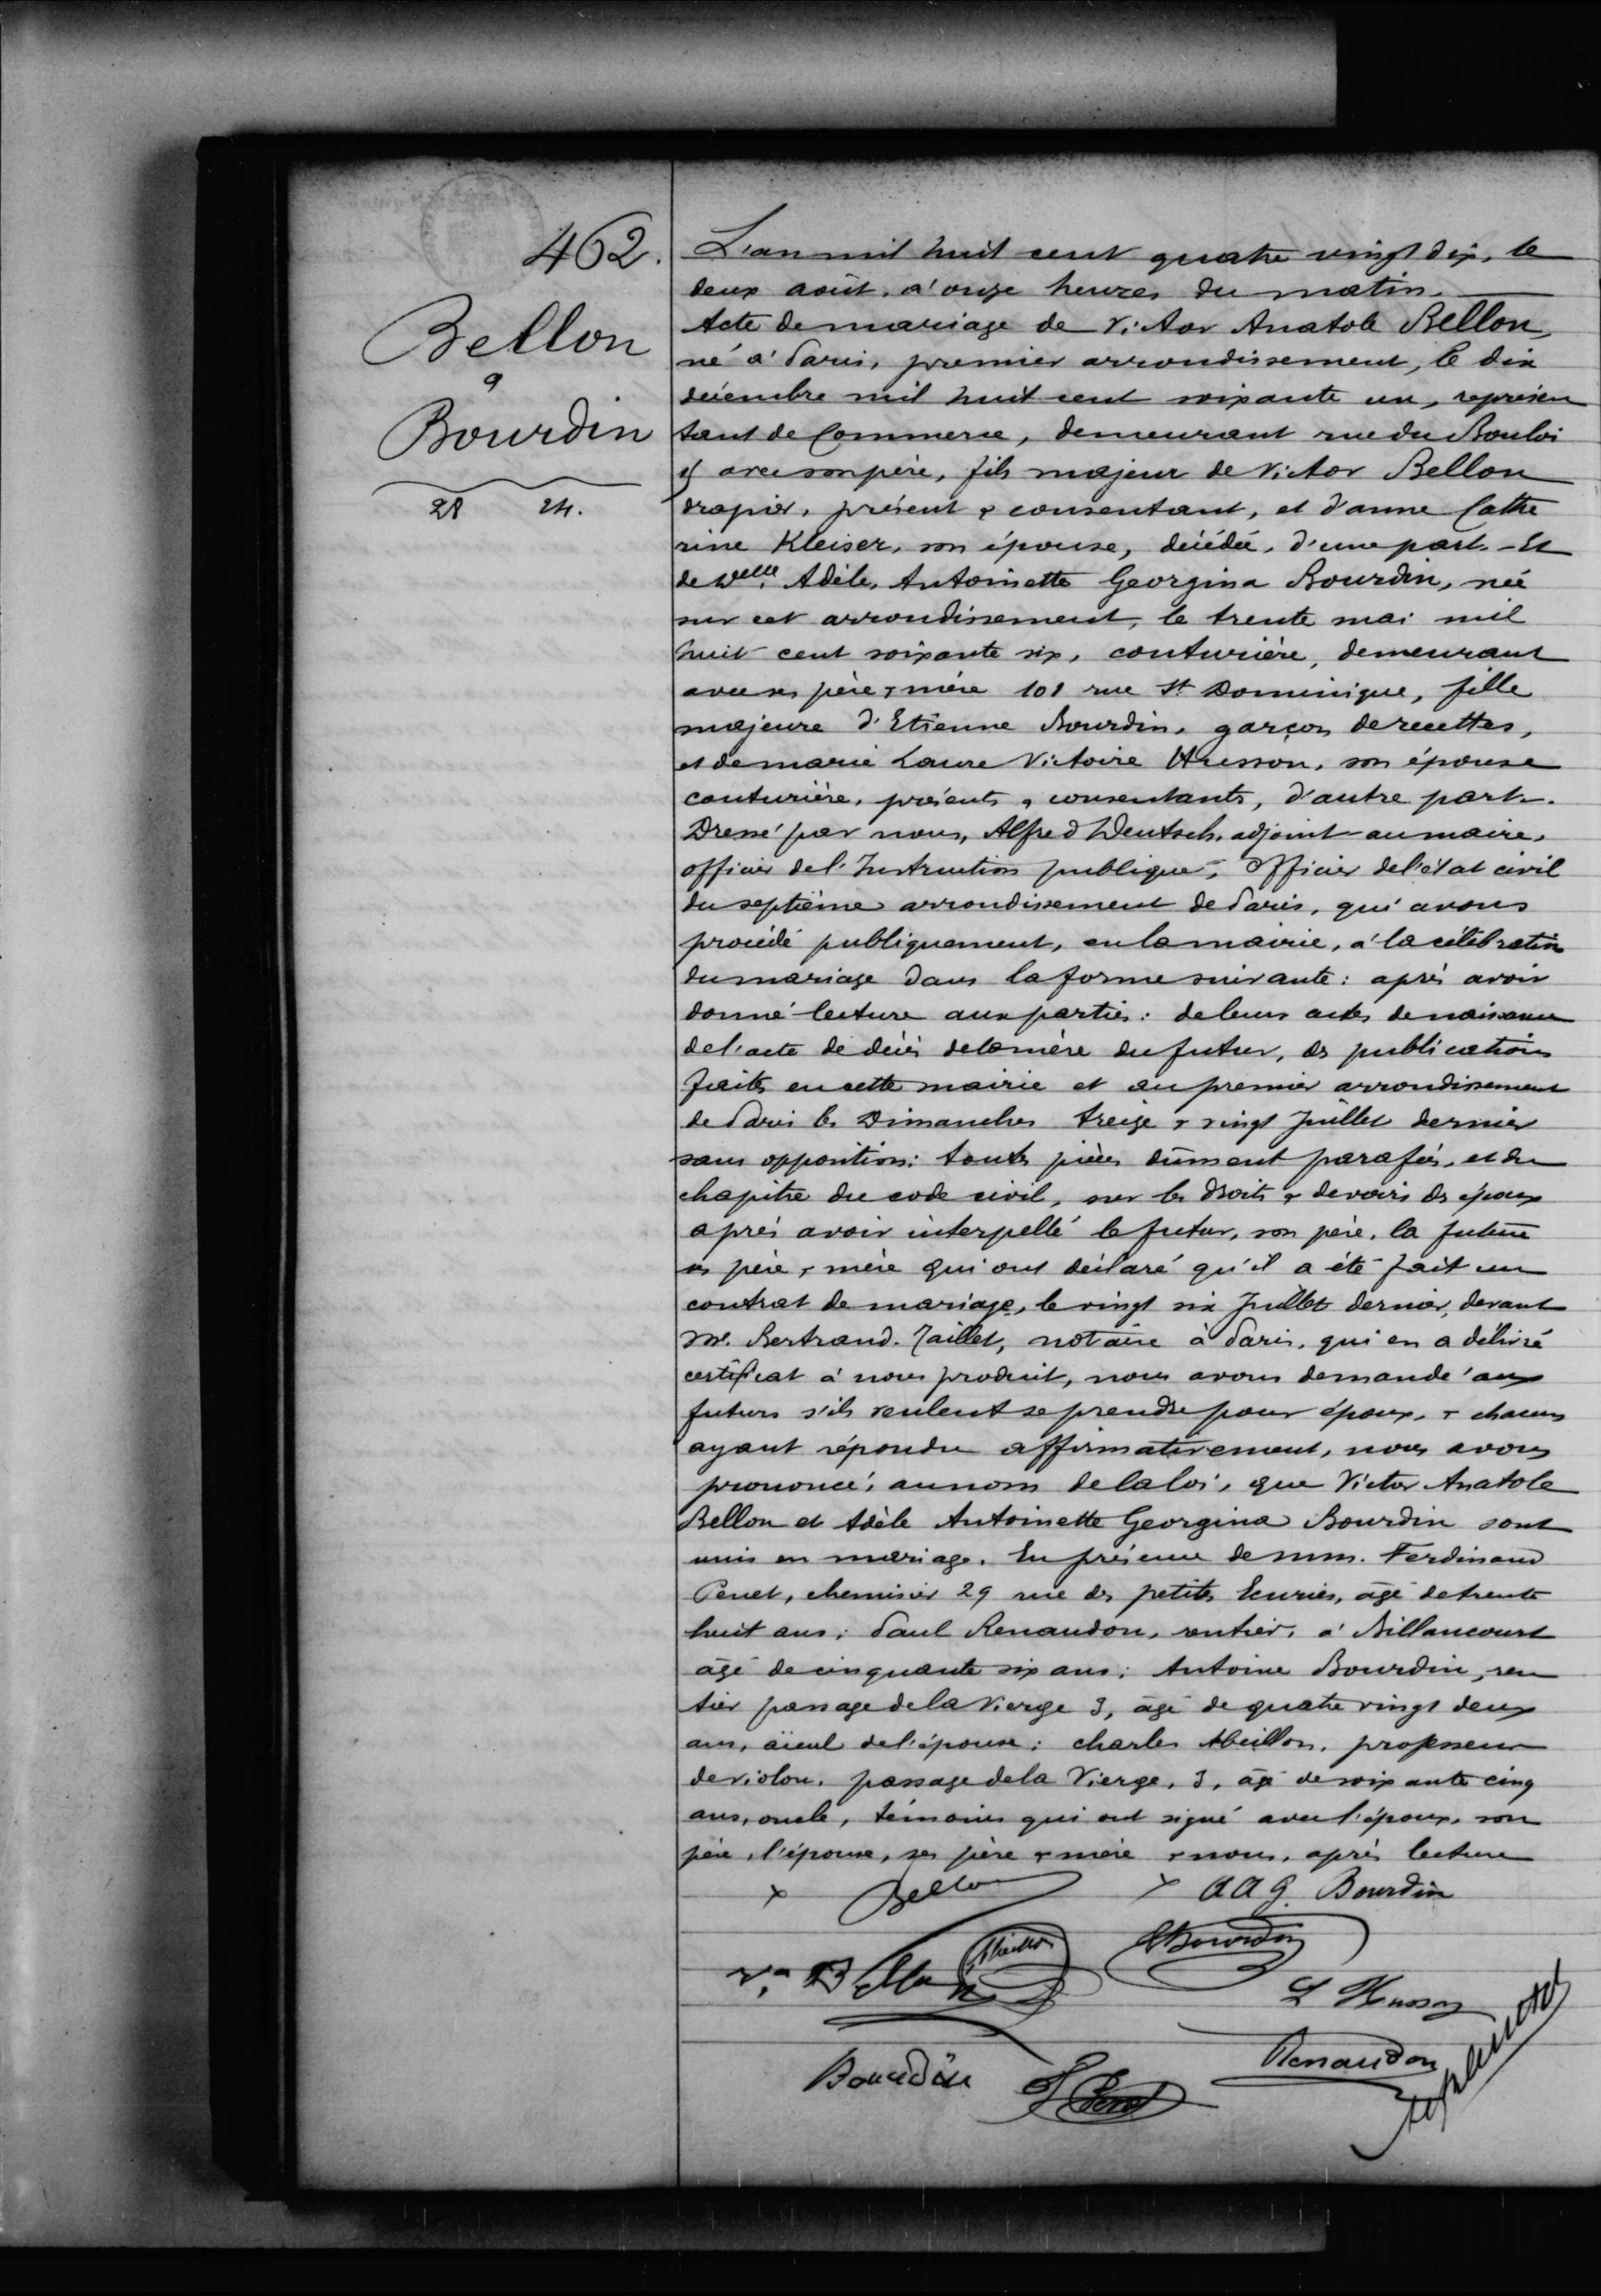462
Bellon
&
Bourdin
28 24
L'an mil huit cent quatre vingt dix, le
deux août à onze heures du matin.-
Acte de mariage de Victor Anatole Rellon,
né à Paris, premier arrondissement, le dix
décembre mil huit cent soixante un, représen
tant de Commerce, demeurant rue du Bouloi
et avec son père, fils majeur de Victor Bellon
drapier, présent & consentant, et d'anne Cathe
rine Kleiser, son épouse, décédée, d'une part.-Et
de Adèle Antoinette Georgina Bourdin, née
sur cet arrondissement, le trente mai mil
huit cent soixante six, couturière, demeurant
avec ses père & mère 108 rue St Dominique, fille
majeure d'Etienne Bourdin, garçon de recettes,
et de marie Laure Victoire Kresson, son épouse
couturière, présents & consentants, d'autre part.
Dressé par nous, Alfred Weutsch, adjoint au maire,
officier de l'Instruction publique: Officier de l'état civil
du septième arrondissement de Paris, qui avons
procédé publiquement, en la mairie, à la célébration
du mariage dans la forme suivante: après avoir
donné lecture aux parties: de leurs actes de naissance
de l'acte de décès de lamère du futur, des publications
faites en cette mairie et du premier arrondissement
de Paris les Dimanches treize et vingt Juillet dernier
sans opposition: toutes pièces dûment parafées, et du
chapitre du code civil, sur les droits et devoirs des époux
après avoir interpellé le futur, son père, la future
ses père et mère qui ont déclaré qu'il a été fait un
contrat de mariage, le vingt six Juillet dernier, devant
Me Berthand Maillet, notaire à Paris, qui en a délivré
certificat à nous produit, nous avons demandé aux
futurs s'ils veulent se prendre pour époux, et chacun
ayant répondu affirmativement, nous avons
prononcé: au nom de la loi, que Victor Anatole
Bellon et Adèle Antoinette Georgina Bourdin sont
unis en mariage. En présence de MM Ferdinand
Cerret, chemisier 29 rue des petits leurier, âgés de trente
huit ans; Paul Renaudon, rentier, à Sillancourt
âgé de cinquante six ans; Antoine Bourdin rue
du passage de la Vierge 9, âgé de quatre vingt deux
ans, aïeul de l'épouse; Charle Abillon, professeur
de violon. passage dela Vierge, 9, âgé de soixante cinq
ans, oncle, témoins qui ont signé avec l'époux, son
père, l'épouse, ses père et mère, après lecture
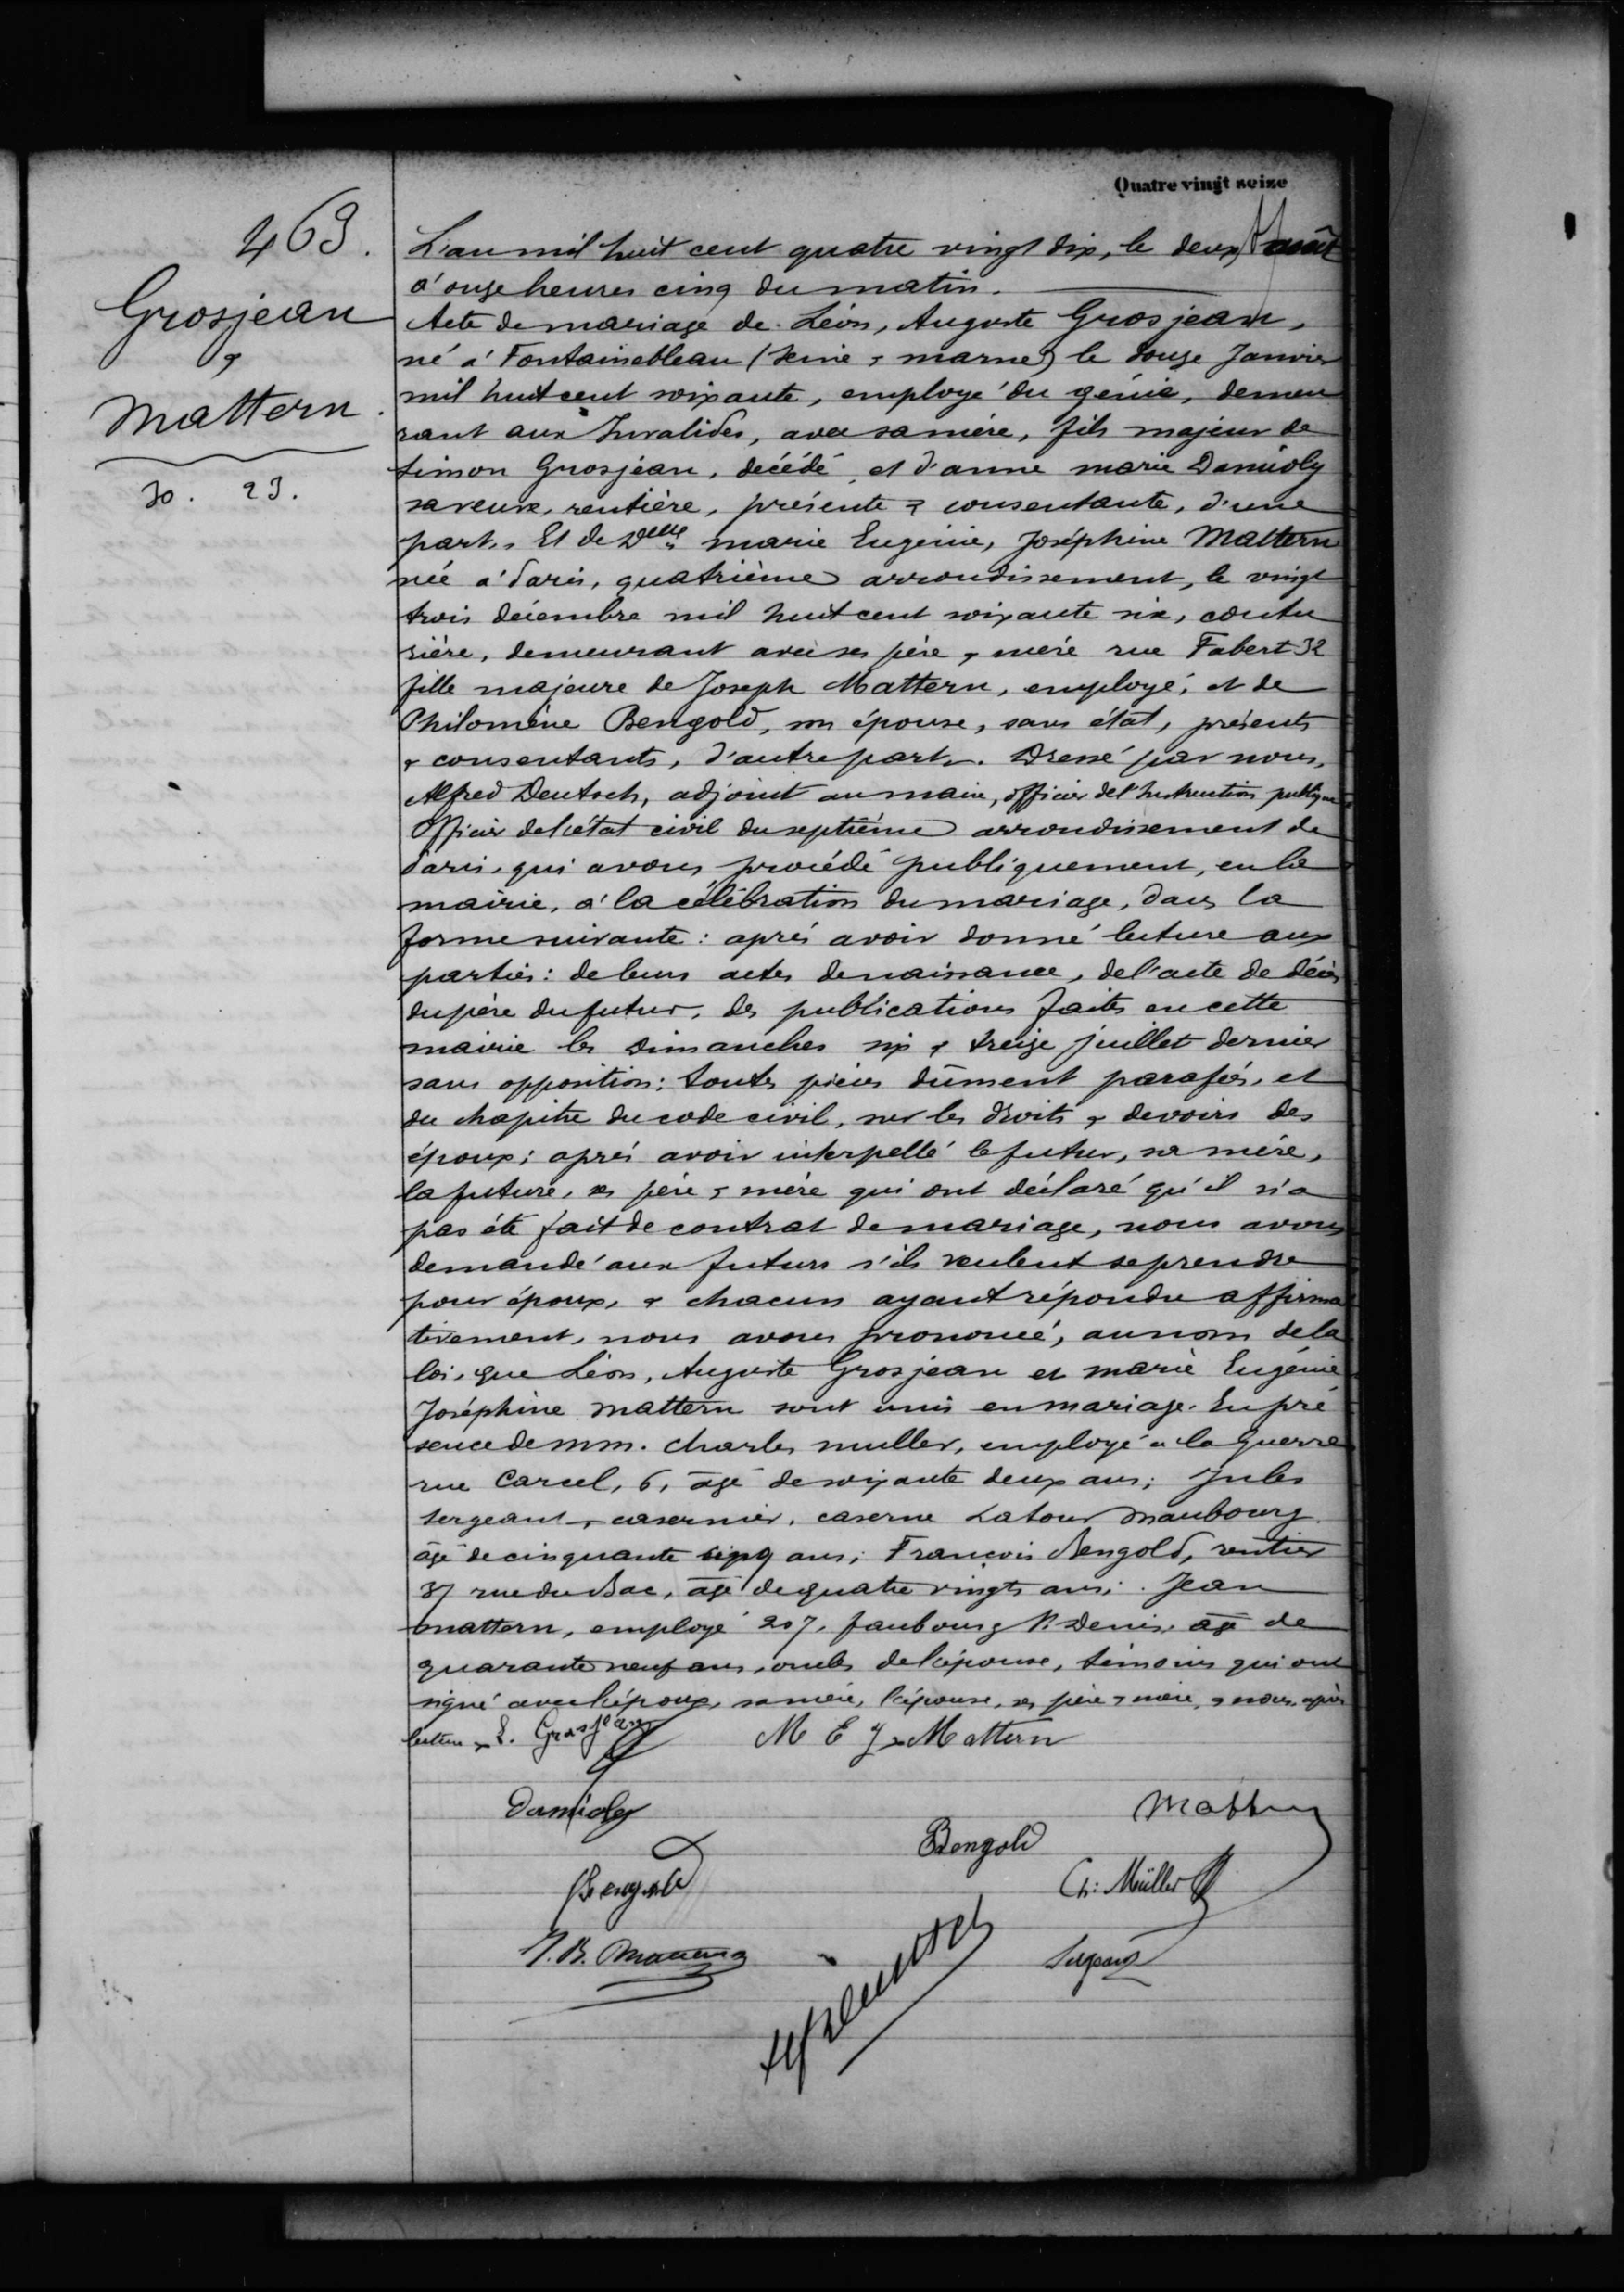469
Grosjean
&
Mattern
30 23
L'an mil huit cent quatre vingt dix, le deux août
à onze heures cinq dumatin. -
Acte de mariage de Léon, Auguste Gros jean,
né à Fontainebleau (Seine & Marne) le douze Janvier
mil huit cent soixante, employé du génie, demeu
rant aux Invalides, avec sa mère, fils majeur de
Simon Grosjean, décédé et d'anne marie Danusly
sa veuve, rentière, présente & consentante, d'une
part. Et d'Anne marie Eugénie, Joséphine Matterne
né à Paris, quatrième arrondissement, le vingt
trois décembre mil huit cent soixante six, coutu
rière, demeurant avec ses père et mère rue Fabert 92
fille majeure de Joseph Mattern, employé, et de
Philomène Bengold, son épouse, sans état, présents
& consentants, d'autre part. Dressé par nous,
Alfred Deutsch, adjoint au maire, officier de l'instruction publique
Officier de l'état civil du septième arrondissement de
Paris, qui avons procédé publiquement, en la
mairie, à la célébration dumariage, dans la
forme suivante: après avoir donné lecture aux
parties: de leurs actes de naissance, del'acte de décès
du père du futur, des publications faites en cette
mairie les dimanches six & treize juillet dernier
sans opposition: toutes pièces dûment paragées, et
du chapitre du code civil, sur les droits & devoirs des
époux; après avoir interpellé lefutur, sa mère,
La future, ses père & mère qui ont déclaré qu'il n'a
pas été fait de contrat demariage, nous avons
demandé aux futurs s'ils veulent se prendre
pour époux, & chacun ayant répondu affirma
tivement, nous avons prononcé; au nom de la
loi, que Léon, Auguste Grosjean et Marie Eugénie
Joséphine Mattern sont unis en mariage. Enpré
sence de mm Charles Muller, employé à la Guerre
rue Carcel, 6, âgé de soixante deux ans; Jules
Sergeant -casernier, caserne Latour Maubourg
âgé de cinquante cinq ans; François Bengold, rentier
37 rue du Bar, âgé de quatre vingt ans, jean
Onattern, employé 207, faubourg St denis, âgé de
quarante neuf ans, oncles de l'épouse, témoins qui ont
signé avec l'époux sa mère, l'épouse, ses père & mère, & nous après
lecture.